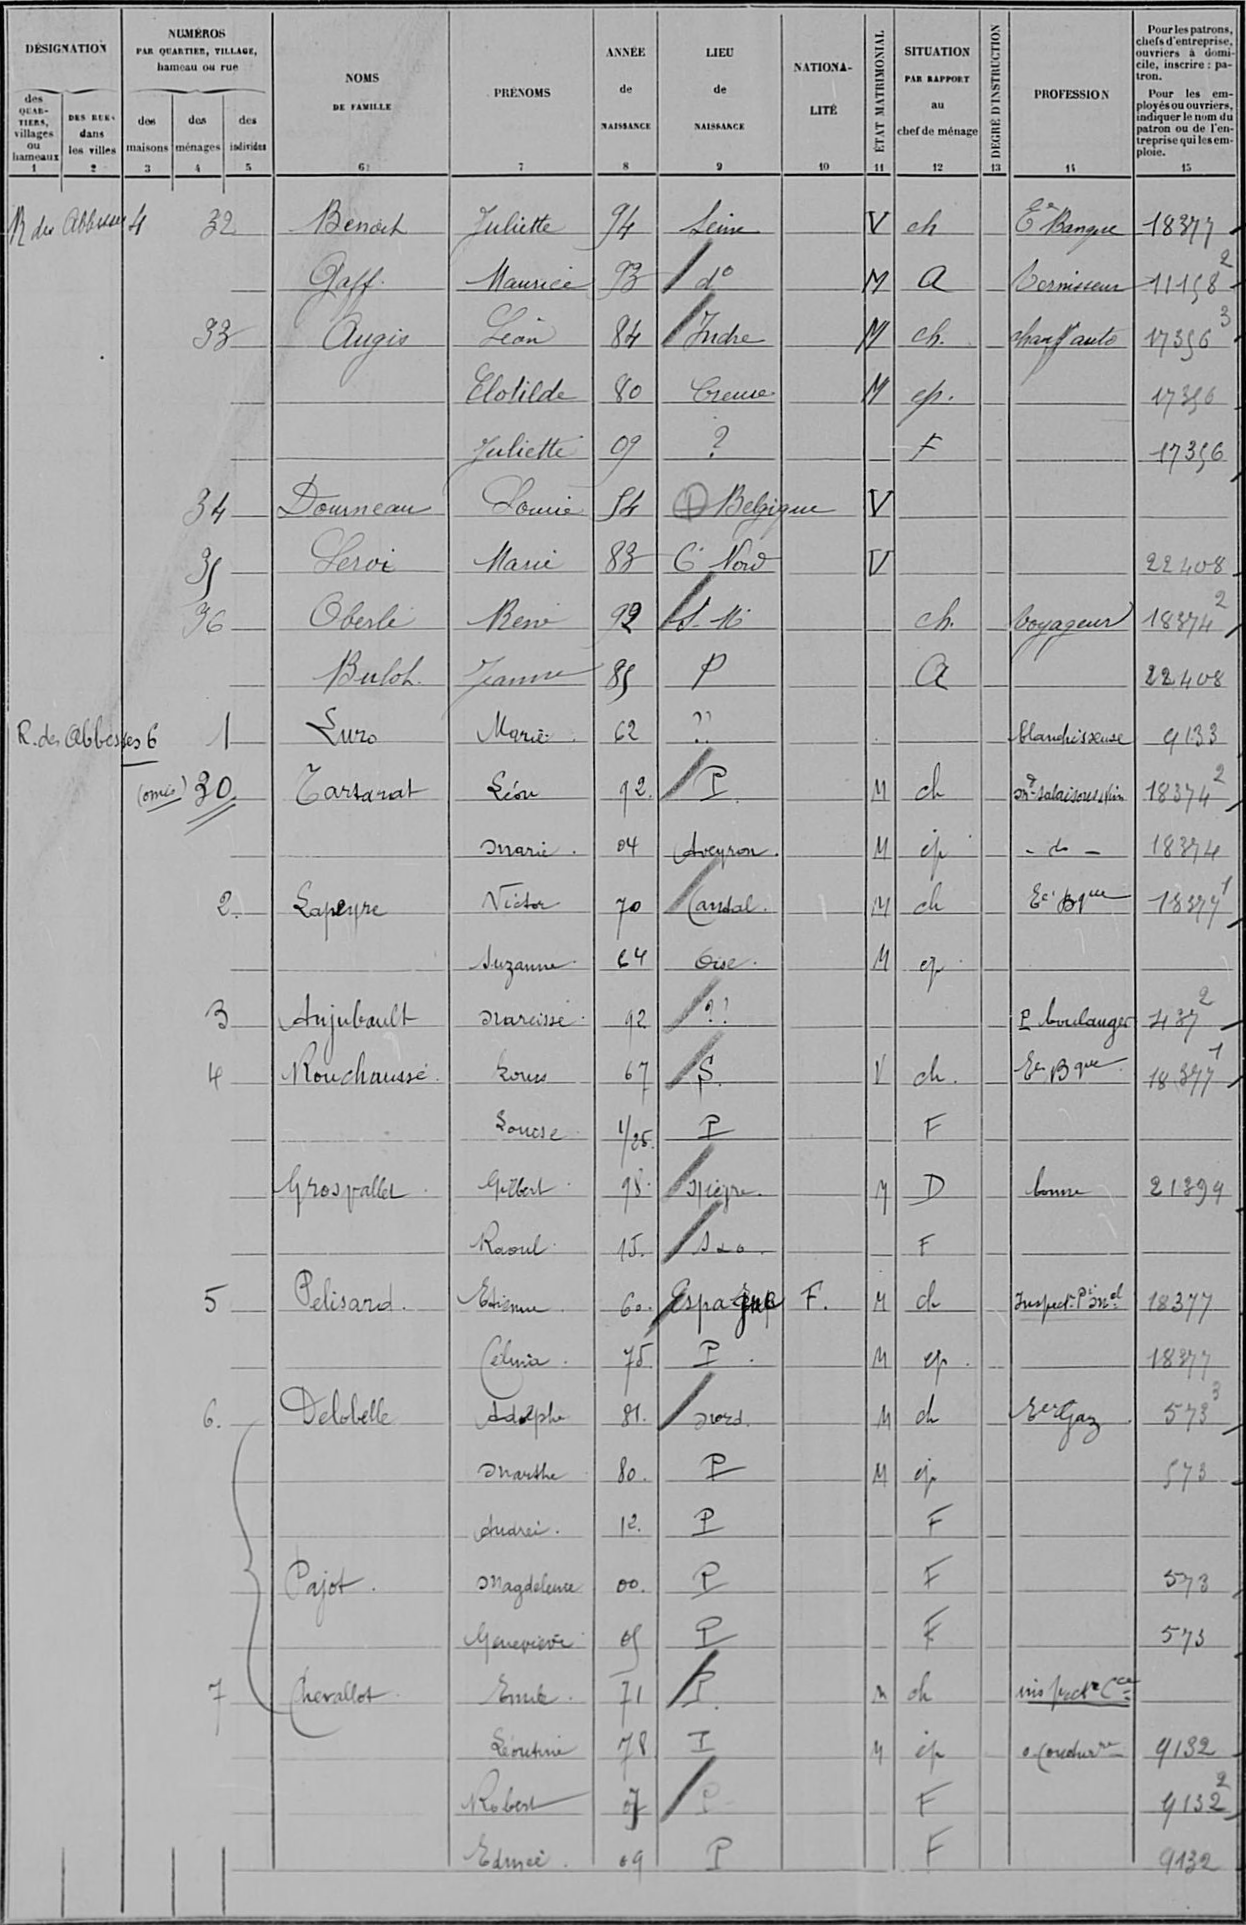Benoit/Juliette/94/Seine/¤/V/ch/¤/E Banque/18377
Gaff/Maurice/93/d°/¤/M/A/¤/Vernisseur/111582
Augis/Léon/84/Indre/¤/M/ch /¤/chauff auto/173563
¤/Clotilde/80/Creuse/¤/M/ep /¤/¤/17356
¤/Juliette/09/¤/¤/¤/F/¤/¤/17356
Dourneau/Lourie/54/Belgique/¤/V/¤/¤/¤/¤
Leroi/Marie/83/C Nord/¤/V/¤/¤/¤/22408
Oberle/René/92/S-M/¤/¤/ch /¤/voyageur/183742
Buloh/Jeanne/85/P/¤/¤/A/¤/¤/22408
Luro/Marie/62/¤/¤/¤/¤/¤/blanchisseuse/9133
Tarsarat/Léon/92/P/¤/M/ch/¤/o-salaisons&vin/183742
¤/marie/04/Aveyron/¤/M/ép/¤/d/18374
Lapeyre/Victor/70/Cantal/¤/M/ch/¤/E Bque/183741
¤/Suzanne/64/Oise/¤/M/ep/¤/¤/¤
Anjubault/Narcisse/92/¤/¤/¤/¤/¤/P boulanger/14372
Rouchaussé/Louis/67/S/¤/V/ch/¤/E Bque/183771
¤/Louise/1 25/P/¤/¤/F/¤/¤/¤
Grosvaller/Gilbert/98/Nièvre/¤/M/D/¤/bonne/21399
¤/Raoult/15/s&o/¤/¤/F/¤/¤/¤
Pelisard/Etienne/60 /Espagne/F /M/ch/¤/Inspect Prinal/18377
¤/Celina/75/P /¤/M/ep/¤/¤/18377
Delobelle/Adolphe/81/Nord/¤/M/ch/¤/EeGaz/5733
¤/Marthe/80/P/¤/M/ep/¤/¤/573
¤/Andrei/12/P/¤/¤/F/¤/¤/¤
Pajot/Magdalena/00 /P/¤/¤/F/¤/¤/573
¤/Geneviève/05/P/¤/¤/F/¤/¤/573
Cherallot/Emile/71/P /¤/m/ch/¤/inspect Cce/¤
¤/Leontine/78/P/¤/M/ép/¤/o couturre/9132
¤/Robert/07/P/¤/¤/F/¤/¤/91322
¤/Edmée/09/P/¤/¤/F/¤/¤/9132
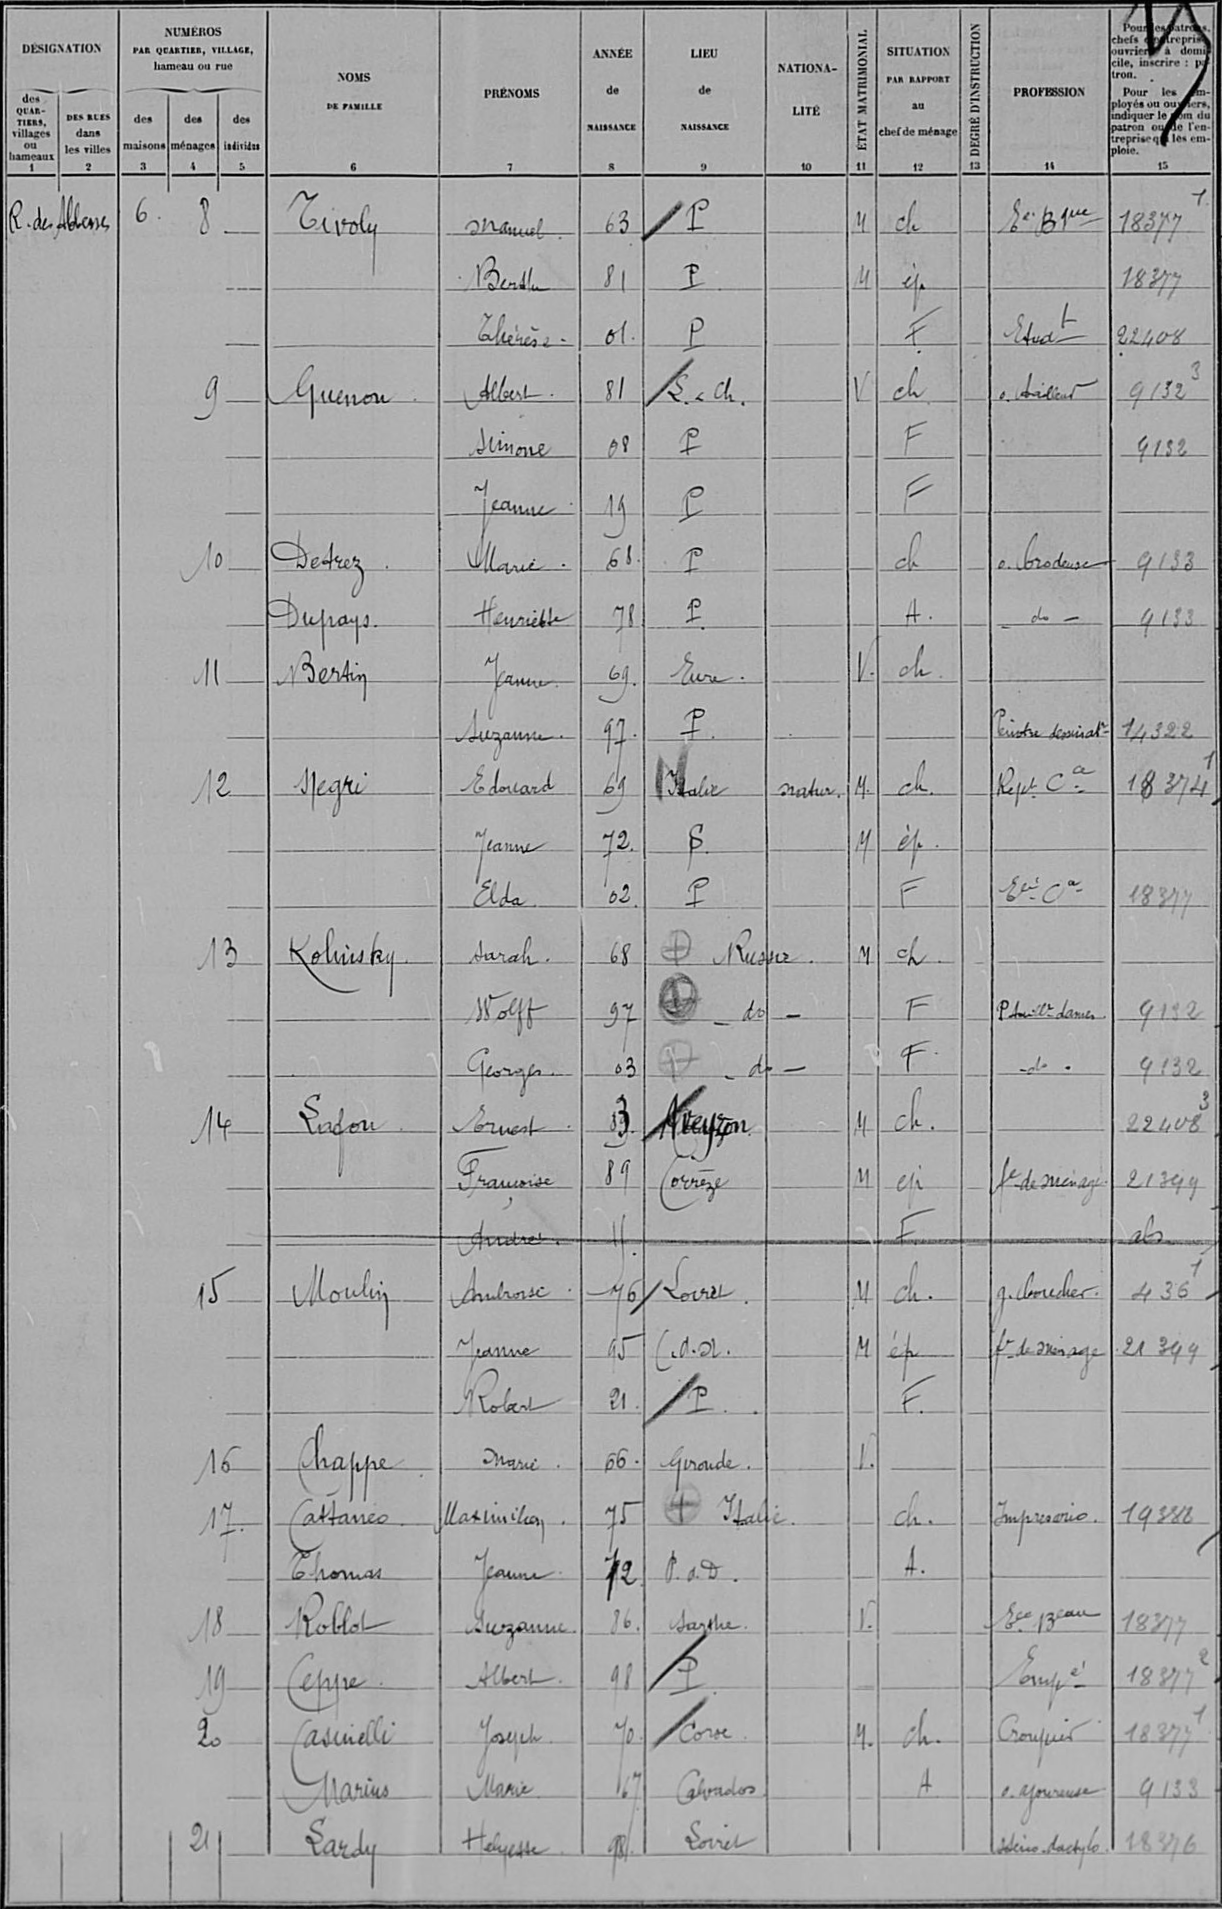Tivoly/Samuel/63/P/¤/M/ch/¤/E Bque/18377
¤/Berthe/81/P/¤/M/ép/¤/¤/18377
¤/Thérèse/01/P/¤/¤/F/¤/Etudt/22408
Guenon/Albert/81/L&CH /¤/V/ch/¤/o tailleur/91323
¤/Simone/08/P/¤/¤/F/¤/¤/4132
¤/Jeanne/19/P/¤/¤/F/¤/¤/¤
Detrez/Marie/68/P/¤/¤/ch/¤/o brodeuse/9133
Duprays/Henriette/78/P/¤/¤/A /¤/d/4133
Bertin/Jeanne/69/Eure/¤/V /ch /¤/¤/¤
¤/Suzanne/97/P /¤/¤/¤/¤/Peintre dessinat/14322
Negri/Edouard/69/Italie/natur/M /ch /¤/Rpt Cce/183741
¤/Jeanne/72/S /¤/M/ép /¤/¤/¤
¤/Elda/02/P/¤/¤/F/¤/E Cce/18377
Kolinsky/sarah/68/Russie/¤/M/ch/¤/¤/¤
¤/Wolf/97/d/¤/¤/F/¤/P taillr dames/9132
¤/Georges/03/d/¤/¤/F/¤/d/4132
Lafon/Ernest/13/Aveyron/¤/M/ch /¤/¤/224083
¤/Françoise/89/Corrèze/¤/M/ep/¤/f de ménage/21399
¤/Andre/11/¤/¤/¤/F/¤/¤/abs
Moulin/Ambroise/76/Loiret/¤/M/ch /¤/g boucher/4361
¤/Jeanne/95/C d or/¤/M/ép/¤/f de ménage/21399
¤/Robert/21/P/¤/¤/F /¤/¤/¤
Chappe/Marie/66/Gironde/¤/I /¤/¤/¤/¤
Cattanes/Maximilien/75/Italie/¤/¤/ch /¤/Impresario/19388
Thomas/Jeanne/72/P d D /¤/¤/A /¤/¤/¤
Roblot/Suzanne/86/Sarthe/¤/V /¤/¤/E Beau/18377
Ceppe/Albert/98/P/¤/¤/¤/¤/Empé/183772
Casinelli/Joseph/70/Corse/¤/M /ch /¤/Croupier/183771
Marius/Marie/67/Calvados/¤/¤/A/¤/o ajoureuse/9133
Lardy/Helyette/98/Loiret/¤/¤/¤/¤/steno dactylo /18376